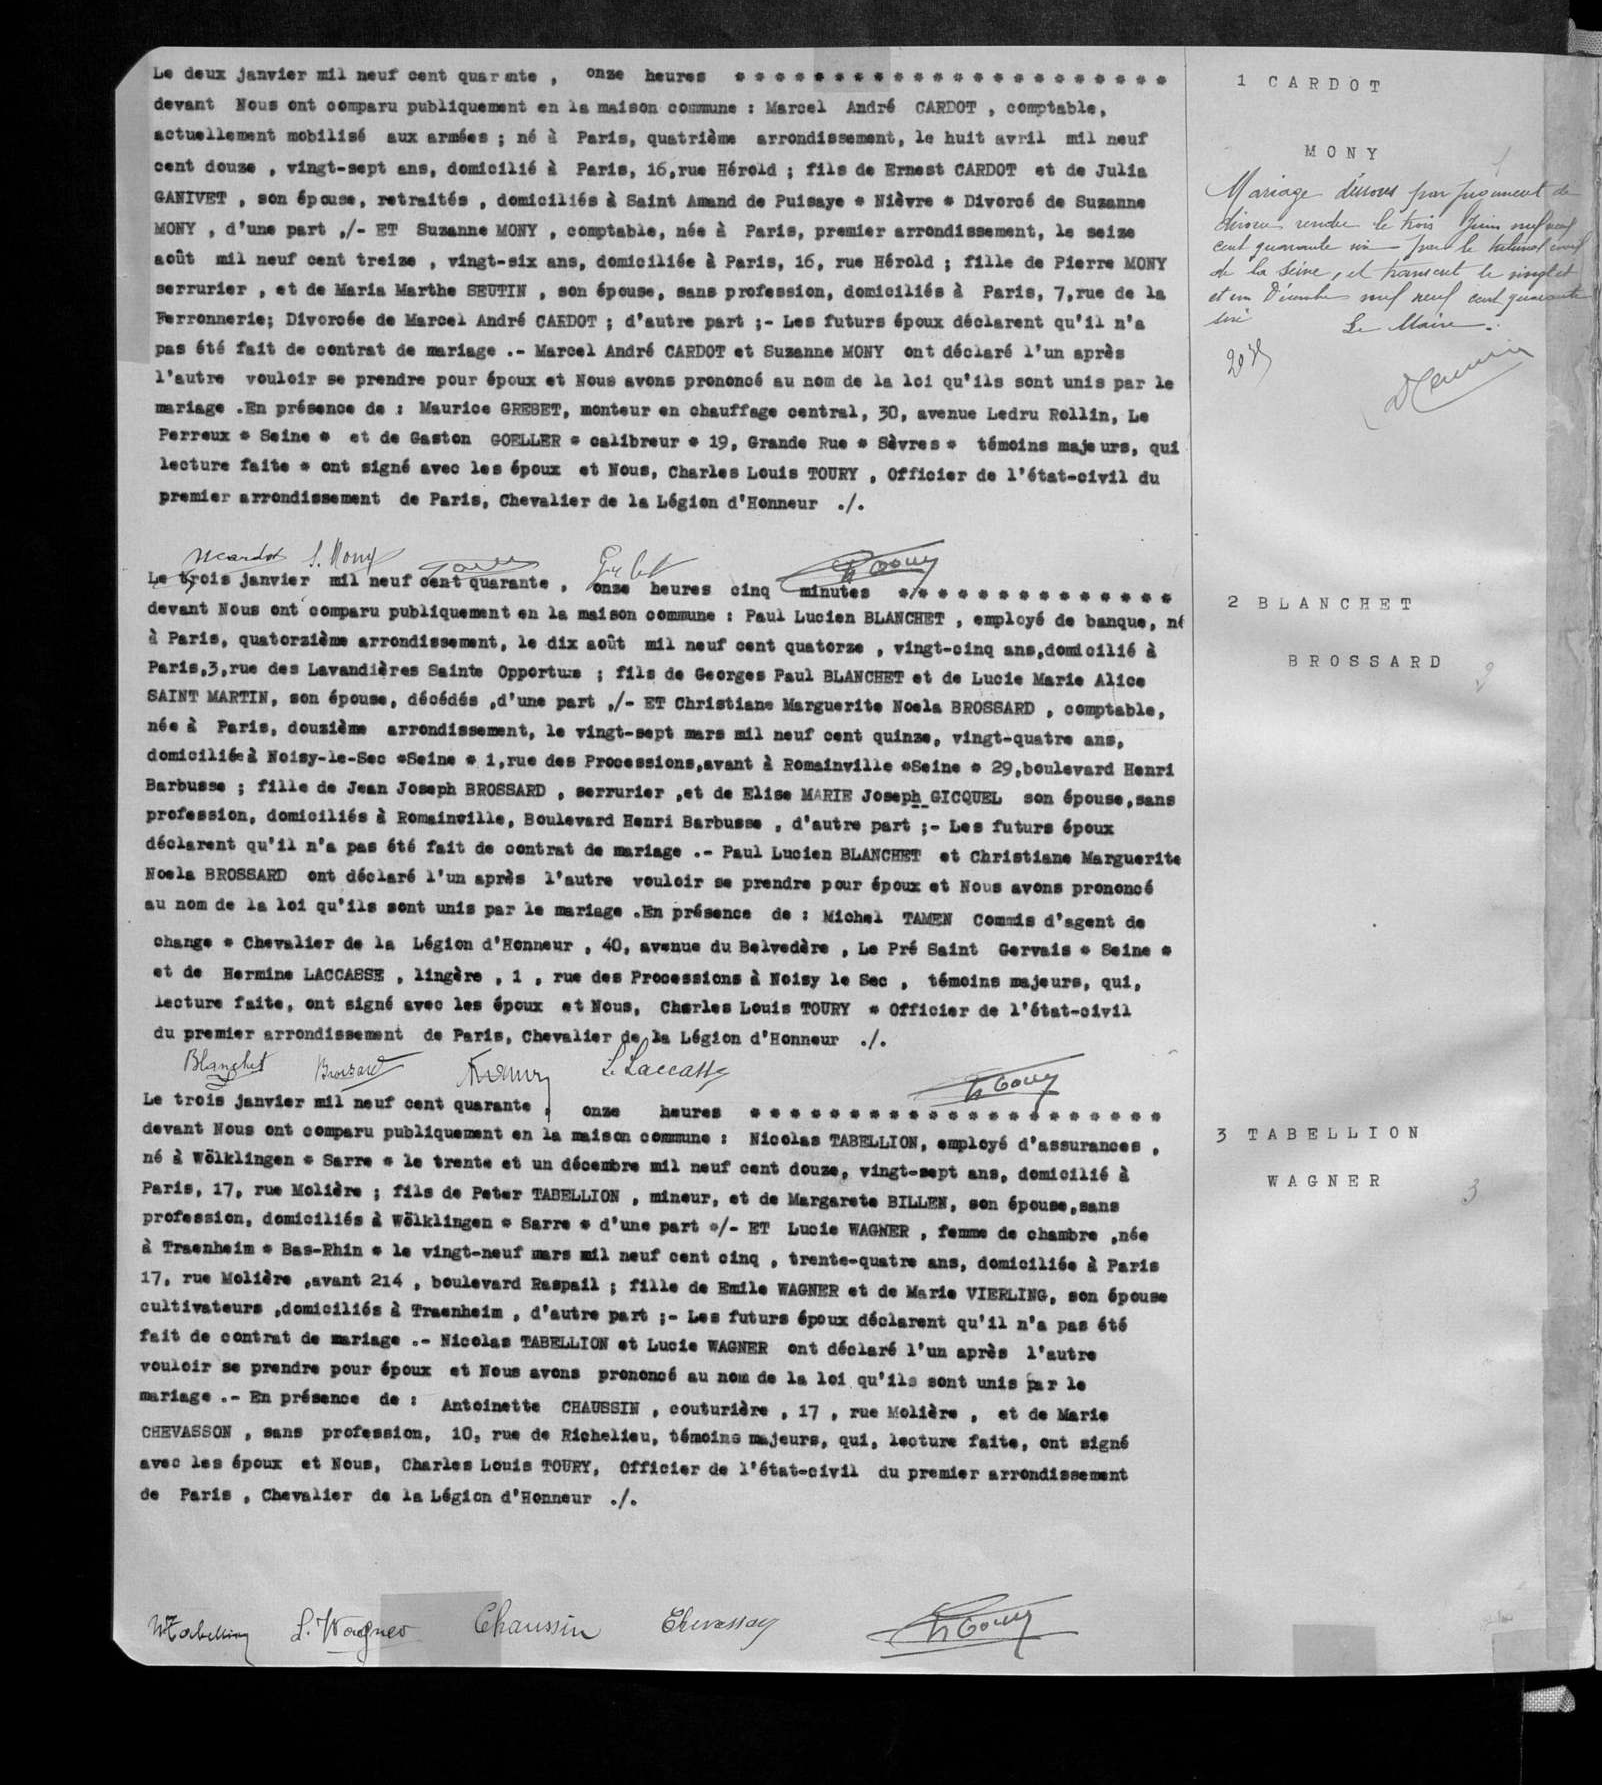Le deux janvier mil neuf cent quarante, onze heure ****
devant Nous ont comparu publiquement en la maison commune: Marcel André CARDOT, comptable,
actuellement mobilisé aux armées; né à Paris, quatrième arrondissement, le huit avril mil neuf
cent douze, vingt-sept ans, domicilié à Paris, 16, rue Hérole: fils de Ernest CARDOT et de Julia
GANIVET, son épouse, retraités, domiciliés à Saint Amand de Puisaye * Nièvre * Divorcé de Suzanne
MONY, d'une part ,/-ET Suzanne MONY, comptable, née à Paris, premier arrondissement, le seize
août mil neuf cent treize, vingt-six ans, domiciliée à Paris, 16, rue Hérold; fille de Pierre MONY
serrurier, et de Maria Marthe SEUTIN, son épouse, sans profession, domiciliés à Paris, 7, rue de la
Ferronerie; Divorcée de Marcel André CAEDOT; d'autre part:-Les futurs époux déclarent qu'il n'a
pas été fait de contrat de mariage .-Marcel André CARDOT et Suzanne MONY ont déclaré l'un après
l'autre vouloir se prendre pour époux, et Nous avons prononcé au Nom de la Loi, qu'ils sont unis par le
mariage. En présence de: Maurice GREBET, monteur en chauffage central, 30, avenue Ledru Rollin, Le
Perreux * Seine * et de Gaston GOELIER * calibreur * 19, Grande Rue * Sèvres * témoins majeurs, qui
lecture faite * ont signé avec les époux et Nous, Charles Louis TOURY, Officier de l'état-civil du
premier arrondissement de Paris, Chevalier de la Hégion d'Honneur.
Le trois janvier mil neuf cent quarante, onze heure cinq minutes ****
devant Nous ont comparu publiquement en la maison commune: Paul Lucien BLANCHET, employé de banque, né
à Paris, quatorzième arrondissement, le dix août mil neuf cent quatorze, vingt-cinq ans, domicilié à
Paris, 3, rue des Lavandières Sainte Opportune; fils de Georges Paul BLANCHET et de Lucie Marie Alice
SAINT MARTIN, son épouse, décédés, d'une part ,/-ET Christiane Marguerite Noela BROSSARD, comptable,
née à Paris, dousième arrondissement, le vingt-sept mars mil neuf cent quinze, vingt-quatre ans,
domiciliée à Noisy-le-Sec * Seine * 1, rue des Processions, avant à Romainville * Seine * 29, boulevard Henri
Barbusse: fille de Jean Joseph BROSSARD, serrurier, et de Elise MARIE Joseph GICQUEL son épouse, sans
profession, domiciliés à Romainville, Boulevard Henri Barbusse, d'autre part ;-Les futurs époux
déclarent qu'il n'a pas été fait de contrat de mariage .-Paul Lucien BLANCHET et Christiane Marguerite
Noela BROSSARD ont déclaré l'un après l'autre vouloir se prendre pour époux et Nous avons prononcé
au nom de la loi qu'ils sont unis par le mariage. En présence de: Michel TAMEN Commis d'agent de
change * Chevalier de légion d'Honneur, 40, avenue du Belvedère, le Pré Saint Gervais * Seine *
et de Hermine LACCASSE, lingère, 1, rue des Processions à Noisy le Sec, témoins majeurs, qui,
lecture faite ont signé avec les époux et Nous, Charles Louis TOURY * Officier de l'état-civil
du premier arrondissement de Paris, Chevalier de la Légion d'Honneur ./.
Le trois janvier mil neuf cent quarante, onze heure ****
devant Nous ont comparu publiquement en la maison commune: Nicolas TABELLION, employé d'assurances,
né à Wölklingen * Sarre * le trente et un décembre mil neuf cent douze, vingt-sept ans, domicilié à
Paris, 17, rue Molière; fils de Peter TABELLION, mineur, et de Margarete BILLEN, son épouse, sans
profession, domiciliés à Wölklingen * Sarre * d'une part */-ET Lucie WAGNER, femme de chambre, née
à Trsenheim * Bas-Rhin * le vingt-neuf mars mil neuf cent cinq, trente-quatre ans, domiciliée à paris
17, rue Molière, avant 214, boulevard Raspail; fille de Emilie WAGNER et de Marie VIERLING, son épouse
cultivateurs, domiciliés à Trsenheim, d'autre part ;-Les futurs époux déclarent qu'il n'a pas été
fait de contrat de mariage .-Nicolas TABELLION et Lucie WAGNER ont déclaré l'un après l'autre
vouloir se prendre pour époux et Nous avons prononcé au nom de la loi qu'ils sont unis par le
mariage .-En présence de: Antoinette CHAUSSIN, couturière, 17, rue Molière, et de Marie
CHEVASSON, sans profession, 10, rue de Richelieu, témoins majeurs, qui, lecture faite, ont signé
avec les époux et Nous, Charles Louis TOURY, Officier de l'état-civil du premier arrondissement
de Paris, Chevalier de la Légion d'Honneur ./.
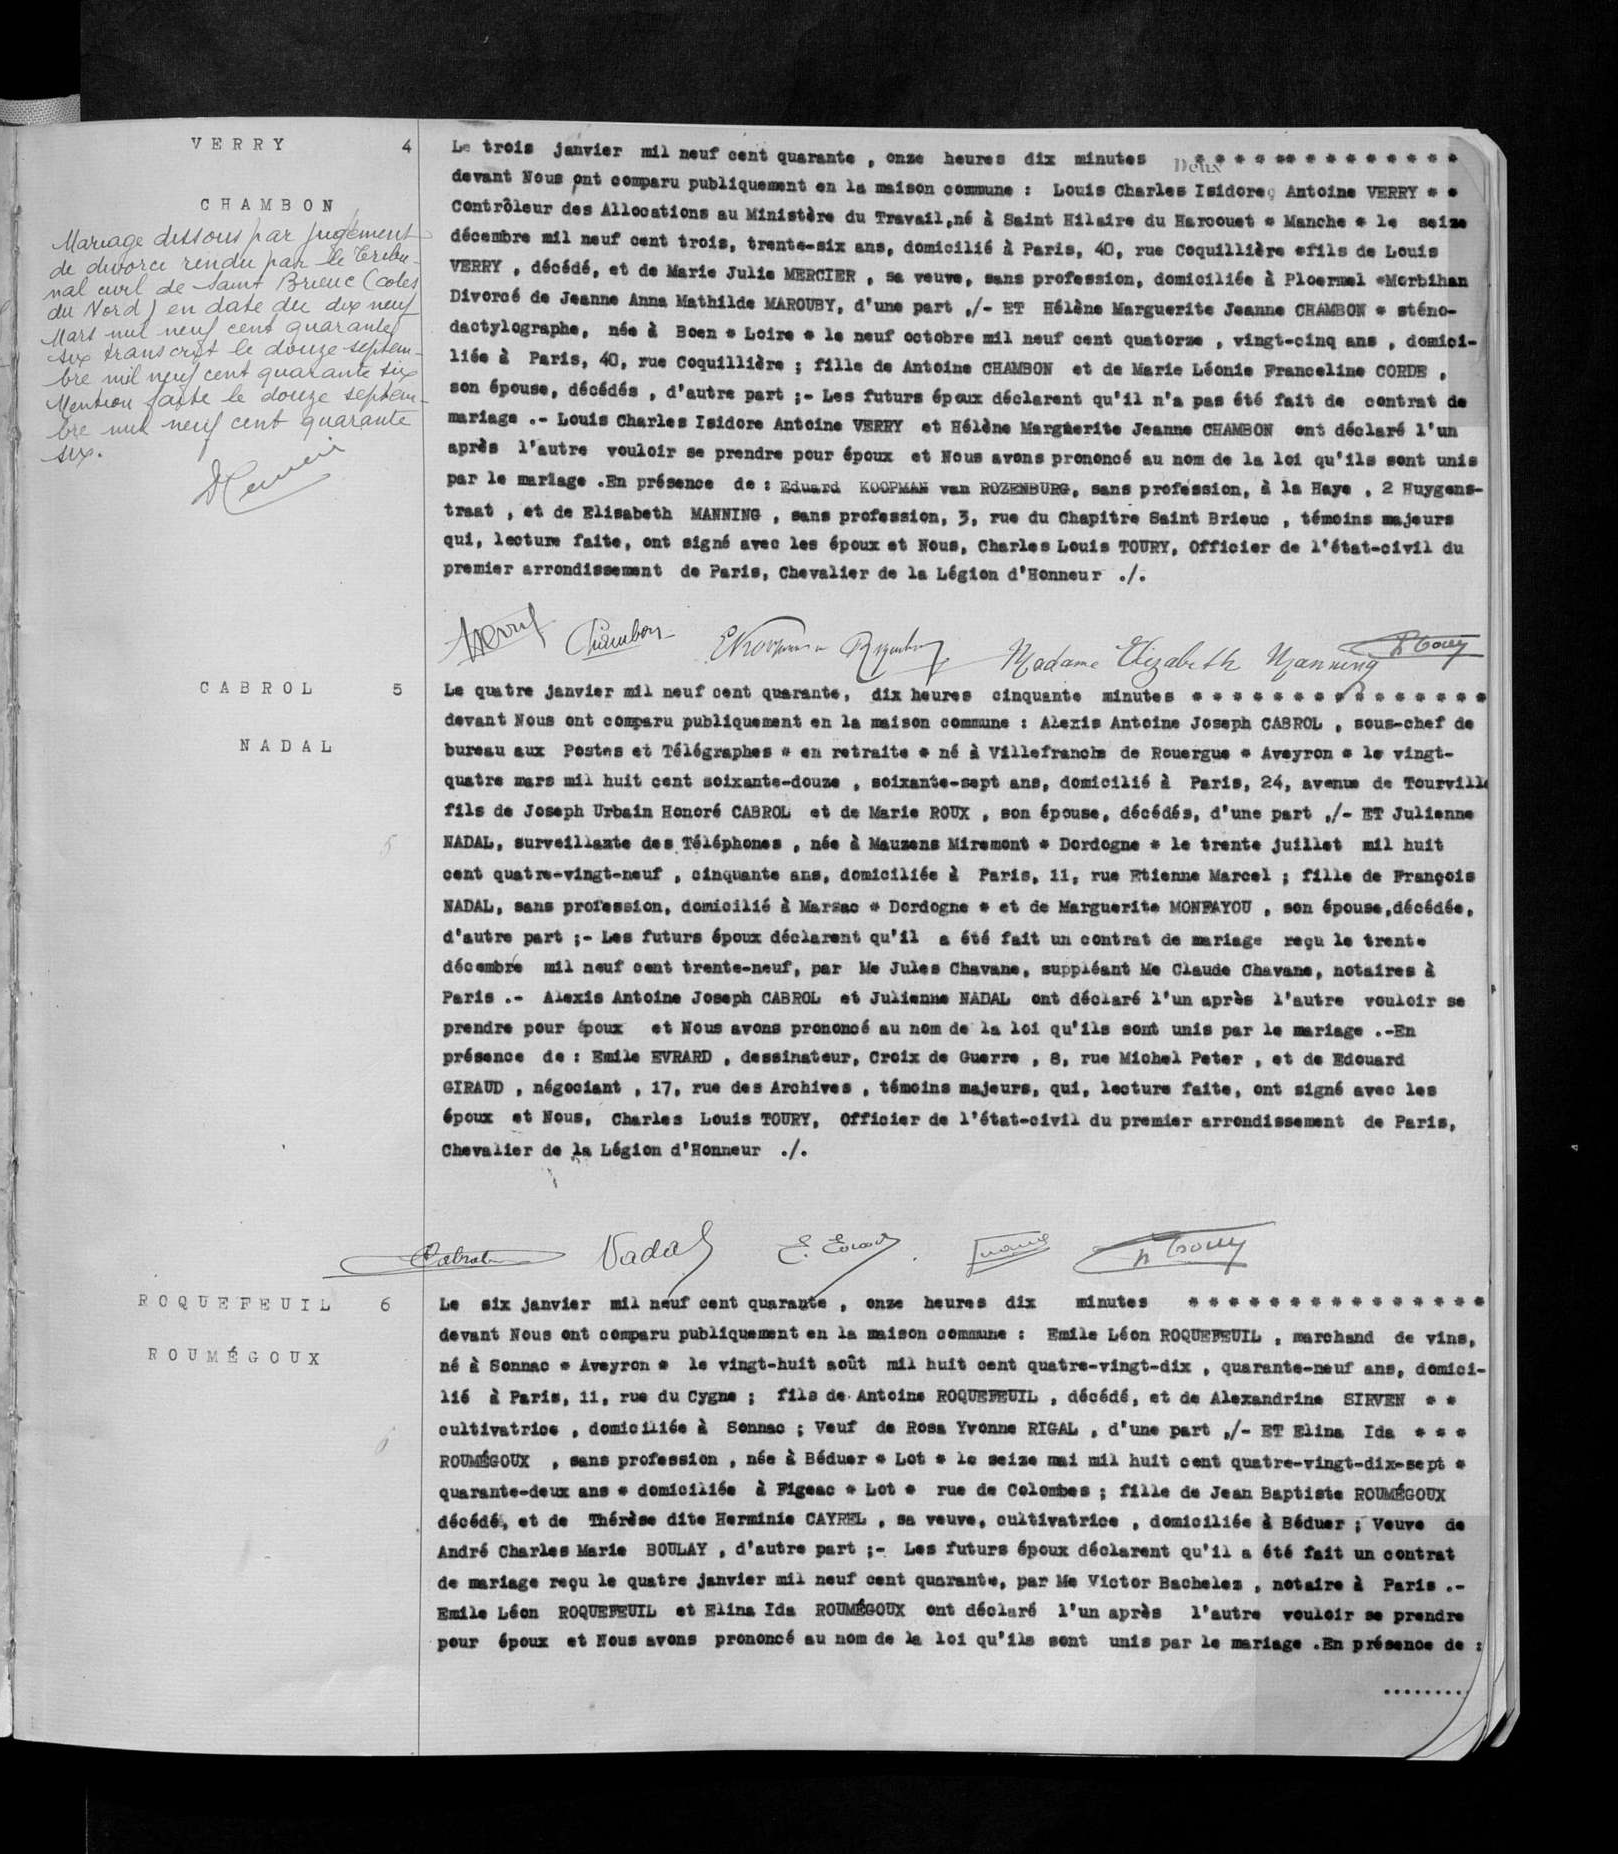Le trois janvier mil neuf cent quarante, onze heures dix minutes ****
devant Nous comparu publiquement en la maison commune: Louis Charles Isidore Antoine VERRY **
Contrôleur des Allocutions au Ministère du Travail, né à Saint Hilaire du Harouet * Manche * le seize
décembre mil neuf cent trois, trente-six ans, domicilié à Paris, 40, rue Coquillière * fils de Louis
VERRY, décédé, et de Marie Julie MERCIER, sa veuve, sans profession, domiciliée) Ploermel * Morbihan
Divorcé de Jeanne Anna Mathilde MAROUBY, d'une part ,/-ET Hélène Marguerite Jeanne CHAMBON * sténo-
dactylographe, née à Boen * Loire * le neuf octobre mil neuf cent quatorze, vingt-cinq ans, domici-
liée à Paris, 40, rue Coquillières; fille de Antoine CHAMBON et de Marie Léonie Franceline CORDE,
son épouse, décédés, d'autre part ;-Les futurs époux déclarent qu'il n'a pas été fait de contrat de
mariage.-Louis Charles Isidore Antoine VERRY et Hélène Marguerite Jeanne CHAMBON ont déclaré l'un
après l'autre vouloir se prendre pour époux et Nous avons prononcé au nom de la loi qu'ils sont unis
par le mariage. En présence de: Eduard KOOPMAN van ROZEMBURG, sans profession, à la Haye, 2 Huygens-
traat, et de Elisabeth MANNING, sans profession, 3, rue du Chapitre Saint Brieux, témoins majeurs
qui, lecture faite, ont signé avec les époux et Nous, Charles Louis TOURY, Officier de l'état-civil du
premier arrondissement de Paris, Chevalier de la Légion d'Honneur ./.
Le quatre janvier mil neuf cent quarante, dix heures cinquante minutes ****
devant Nous ont comparu publiquement en la maison commune: Alexis Antoine Joseph CABROL, sous-chef de
bureau aux Postes et Télégraphes * en retraite * né à Villefranche de Rouergue * Aveyron * le vingt-
quatre mars mil huit cent soixante-douze, soixante-sept ans, domicilié à Paris, 24, avenue de Tourville
fils de Joseph Urbain Honoré CABROL et Marie ROUX, son épouse, décédés, d'une part ./-ET Julienne
NADAL, surveillante des Téléphones, née à Mausent Miromont * Dordogne * le trente juillet mil huit
cent quatre-vingt-neuf, cinquante ans, domiciliée à Paris, 11, Rue Etienne Marcel; fille de François
NADAL, sans profession, domicilié à Marsac *Dordogne* et de Marguerite MONPAYOU, son épouse, décédée,
d'autre part ;-Les futures époux déclarent qu'il a été fait un contrat de mariage reçu le trente
décembre mil neuf cent trente-neuf, par Me Jules Chavane, suppléant Me Claude Chavane, notaires à
Paris .-Alexis Antoine Joseph CABROL et Julienne NADAL ont déclaré l'un après l'autre vouloir se
prendre pour époux et Nous avons prononcé au nom de la loi qu'ils sont unis par le mariage .-En
présence de: Emile EVRARD, dessinateur, Croix de Guerre, 8, rue Michel Peter, et de Edouard
GIRAUD, négociant, 17, rue des Archives, témoins majeurs, qui, lecture faite, ont signé avec les
époux et Nous, Charles Louis TOURY, Officier de l'état-civil du premier arrondissement de Paris,
Chevalier de la Légion d'Honneur ./.
Le six janvier mil neuf cent quarante, onze heures dix minutes ****
devant Nous ont comparu publiquement en la maison commune: Emile Léon ROQUEFEUIL, marchand de vins,
né à Sennac * Aveyron * le vingt-huit août mil huit cent quatre-vingt-dix, quarante neuf ans, domici-
lié à Paris, 11, rue du Cygne: fils de Antoine ROQUEFEUIL, décédé et de Alexandrine SIRVEN **
cultivatrice, domicilié à Sennac; Veuf de rosa Yvonne RIGALE, d'une part ,/-Et Elina Ida ***
ROUMEGOUX, sans profession, née à Béduer * Lot * le sein mai mil huit cent quatre-vingt-dix-sept *
quarante-deux ans * domiciliée à Figeace * Lot * rue de Colombes; fille de Jean Baptiste ROUMEGOUX
décédé, et de Thérèse dite Herminie CAYREL, sa veuve, cultivatrice, domiciliée à Béduer: Veuve de
André Charles Marie BOULAY, d'autre part ;-Les futurs époux déclarent qu'il a été fait un contrat
de mariage reçu le quatre janvier mil neuf cent quarante, par Me Victore Bacheles, notaire à Paris.-
Emilie Léon ROQUEFEUIL et Eline IDA ROUMEGOUX ont déclaré l'un après l'autre vouloir se prendre
pour époux et Nous avons prononcé au nom de la loi qu'ils sont unis ar le mariage. En présence de: